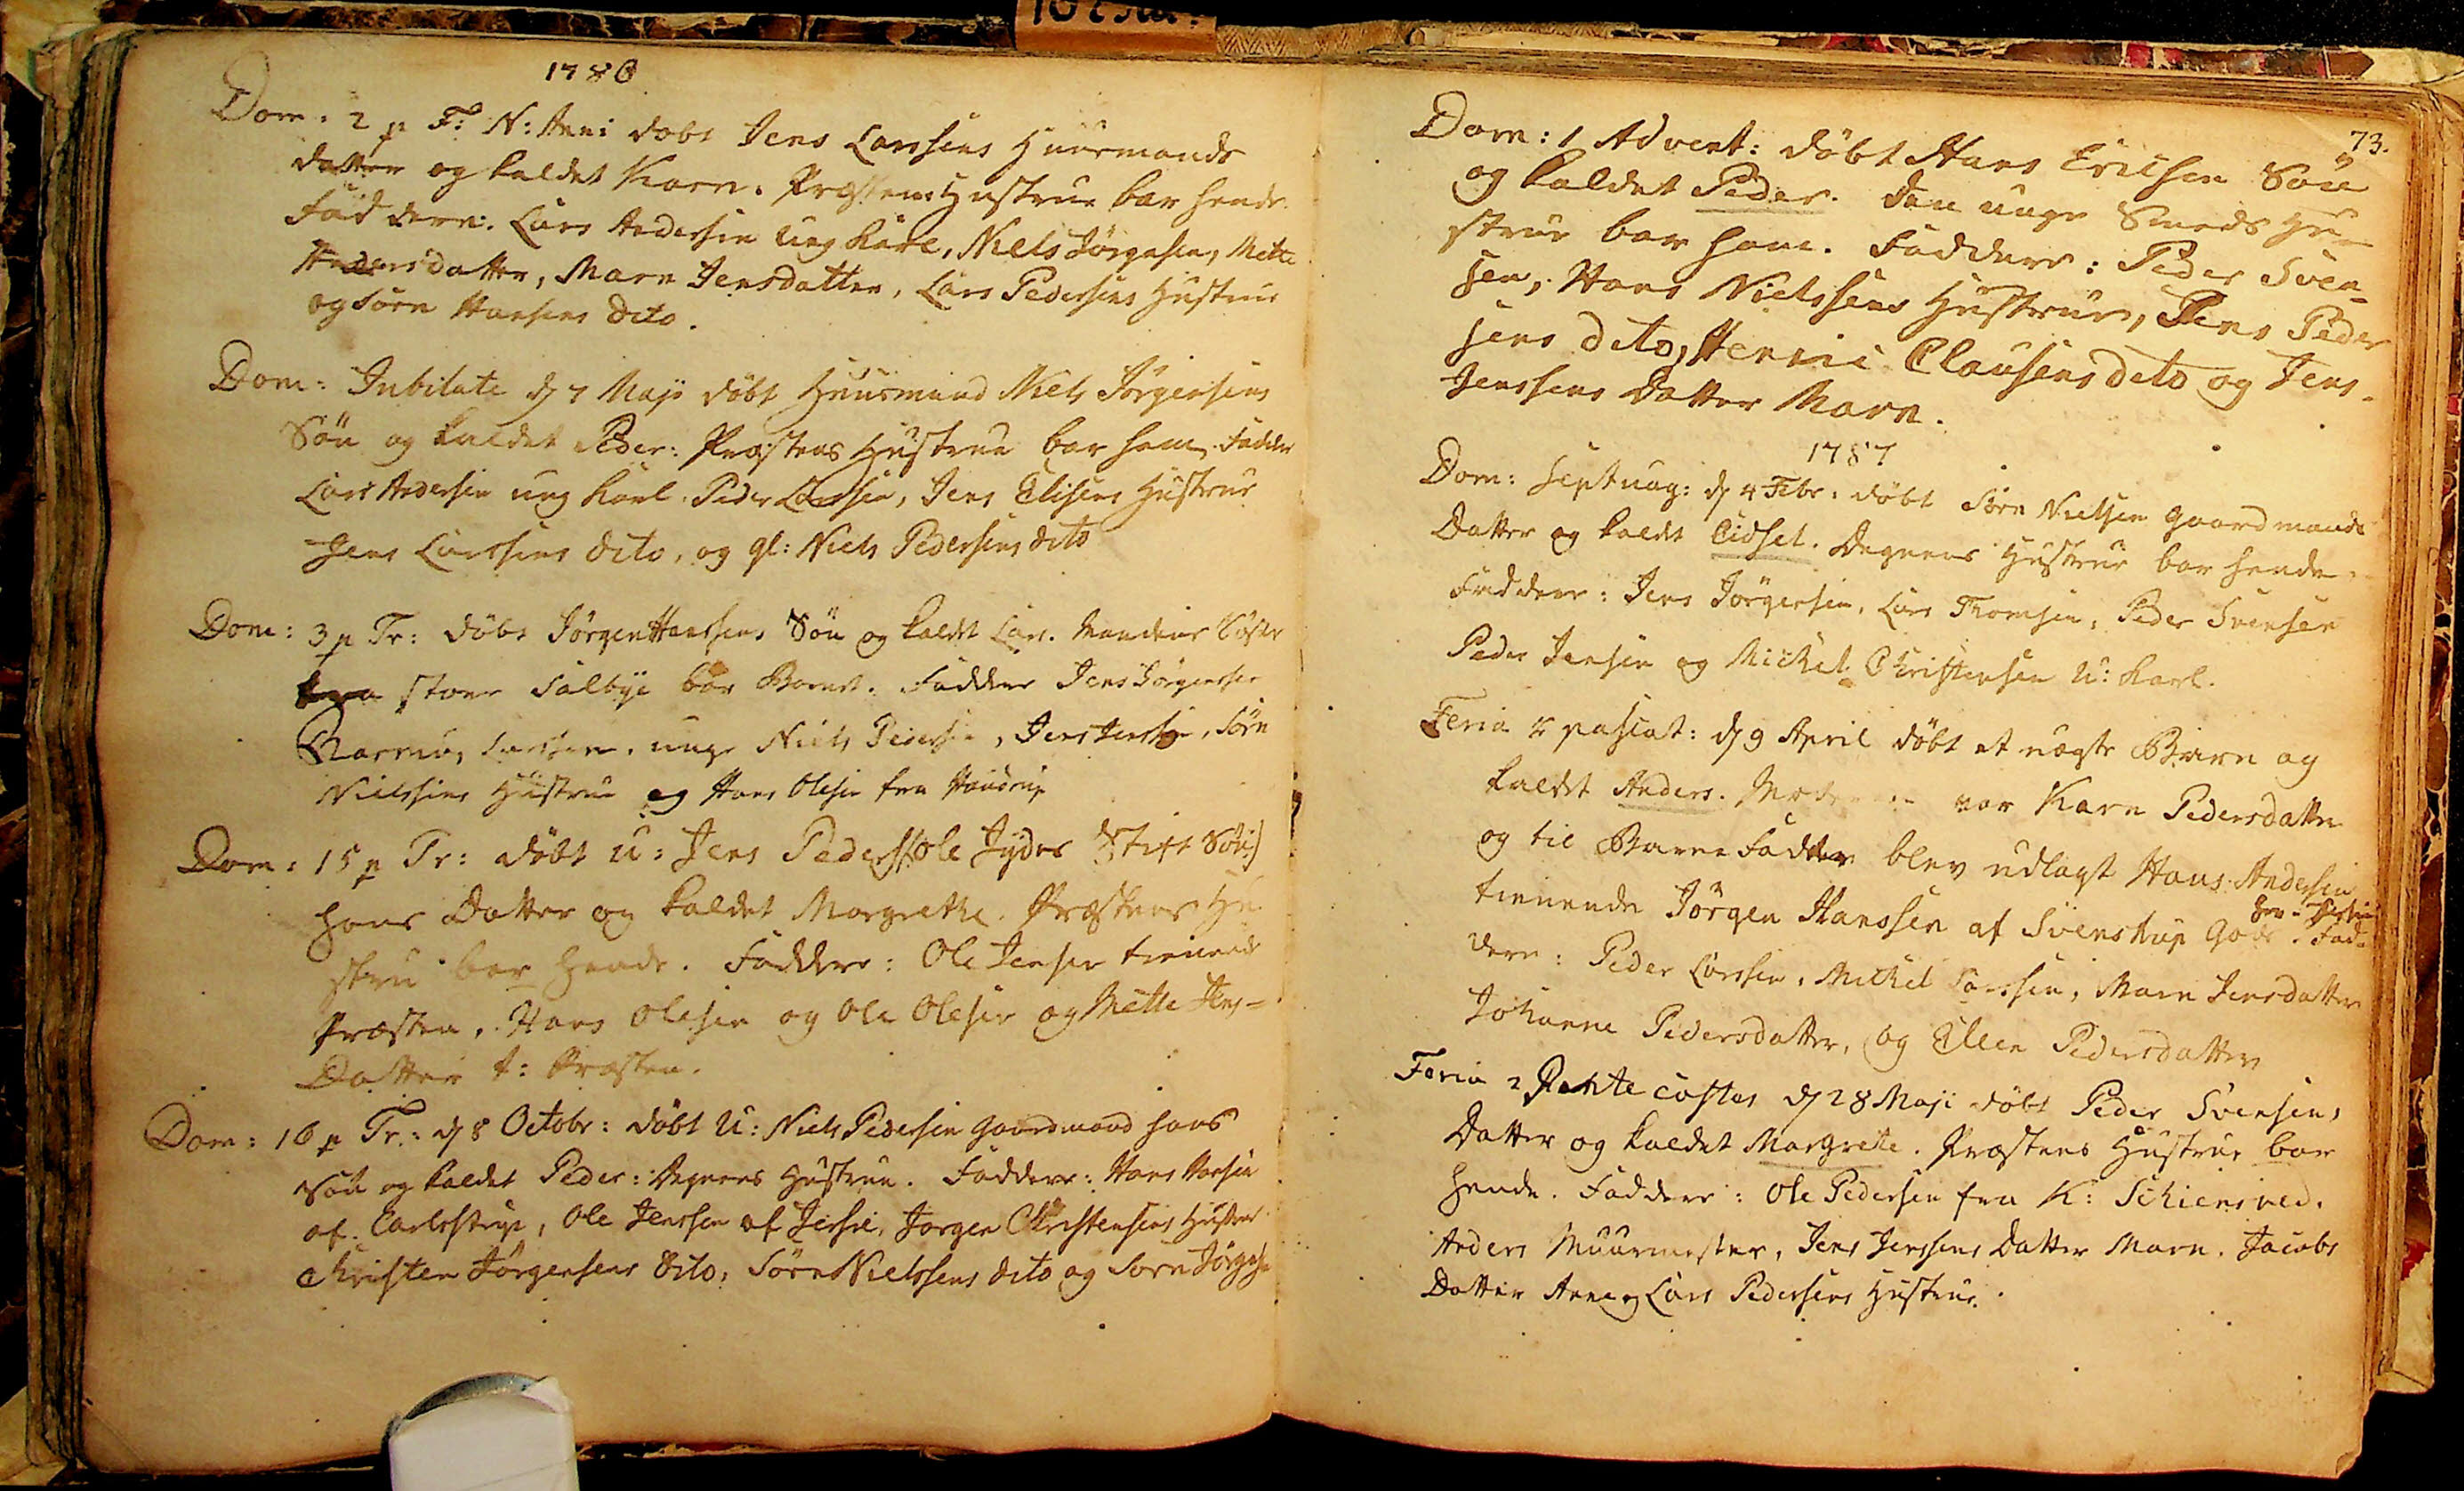1786
Dom: 2 p F: N: Anni døbt Jens Larssens Huusmands
datter og kaldet Karn. Præstens Hustrue bar hende.
Faddere: Lars Andersen Ung Karl, Niels Jørgnsen, Mette
Andersdatter, Marn Jensdatter, Lars Pedersens Hustrue
og Sørn Hansens dito.
Dom: Jubilate d 7 Maji døbt Huusmand Niels Jørgensens
Søn og kaldet Peder: Præstens Hustrue bar ham. Faddere
Lars Andersen ung Karl, Peder Larssen, Jens Elisens Hustrue
Jens Larssens dito, og gl: Niels Pedersens dito
Dom: 3 p Tr: døbt Jørgen Hansens Søn og kaldt Lars. Mandens Søster
fra store Salbye bar Barnet. Faddere Jens Jørgensen
Rasmus Larssen, unge Niels Pedersen, Jens Jenssen, Sørn
Nielssens Hustrue og Hans Olesen fra Haudrup
Dom: 15 p Tr: døbt U: Jens Peders /: Ole Jydes Stift Søn:/
hans Datter og kaldet Margrethe. Præstens Hu
stru bar hende. Faddre: Ole Jensen tienende
Præsten. Hans Olesen og Ole Olesen og Mette Jens¬
Datter t: Præsten.
Dom: 16 p Tr: d 8 Octobr: døbt U: Niels Pedersen Gaardmand hans
Søn og kaldet Peder: Degnens Hustrue. Faddere: Hans Hansen
af Carlstrup, Ole Jenssen af Jersie, Jørgen Christensens Hustrue
Christen Jørgensens dito, Sørn Nielssens dito og Sørn Jørgnsen
73.
Dom: 1 Advent: døbt Hans Ericsen Søn
og kaldet Peder. Den unge Smeds Hu¬
strue bar ham. Faddere: Peder Sven¬
sen; Hans Nielssens Hustrue, Jens Peder
sens dito, Henric Clausens dito og Jens
Jenssens Datter Marn.
1787
Dom: Septuag: d 4 Febr: døbt Sørn Nielsen Gaardmands
Datter og kaldt Cidsel. Degnens Hustrue bar hende.
Faddere: Jens Jørgensen, Lars Thomsen, Peder Svensen
Peder Jensen og Michel Christensen U:Karl.
Feria 2 pascat: d 9 April døbt et uægte Barn og
kaldet Anders. Moderen var Karn Pedersdatter
og til Barne Faddr blev udlagt Hans Andersen
her i Jersie
tienende Jørgen Hanssen af Svenstrup Gods. Fad¬
dere: Peder Larssen, Michel Larssen, Maren Jensdatter
Johanne Pedersdatter, og Ellen Pedersdatter.
Feria 2 Pentecostes d 28 Maji døbt Peder Svensens
Datter og kaldet Margrete. Præstens Hustrue bar 
hende. Faddere: Ole Pedersen fra K: Schiensved.
Anders Muurmester, Jens Jenssens Datter Marn, Jacobs
Datter Anne og Lars Pedersens Hustrue.